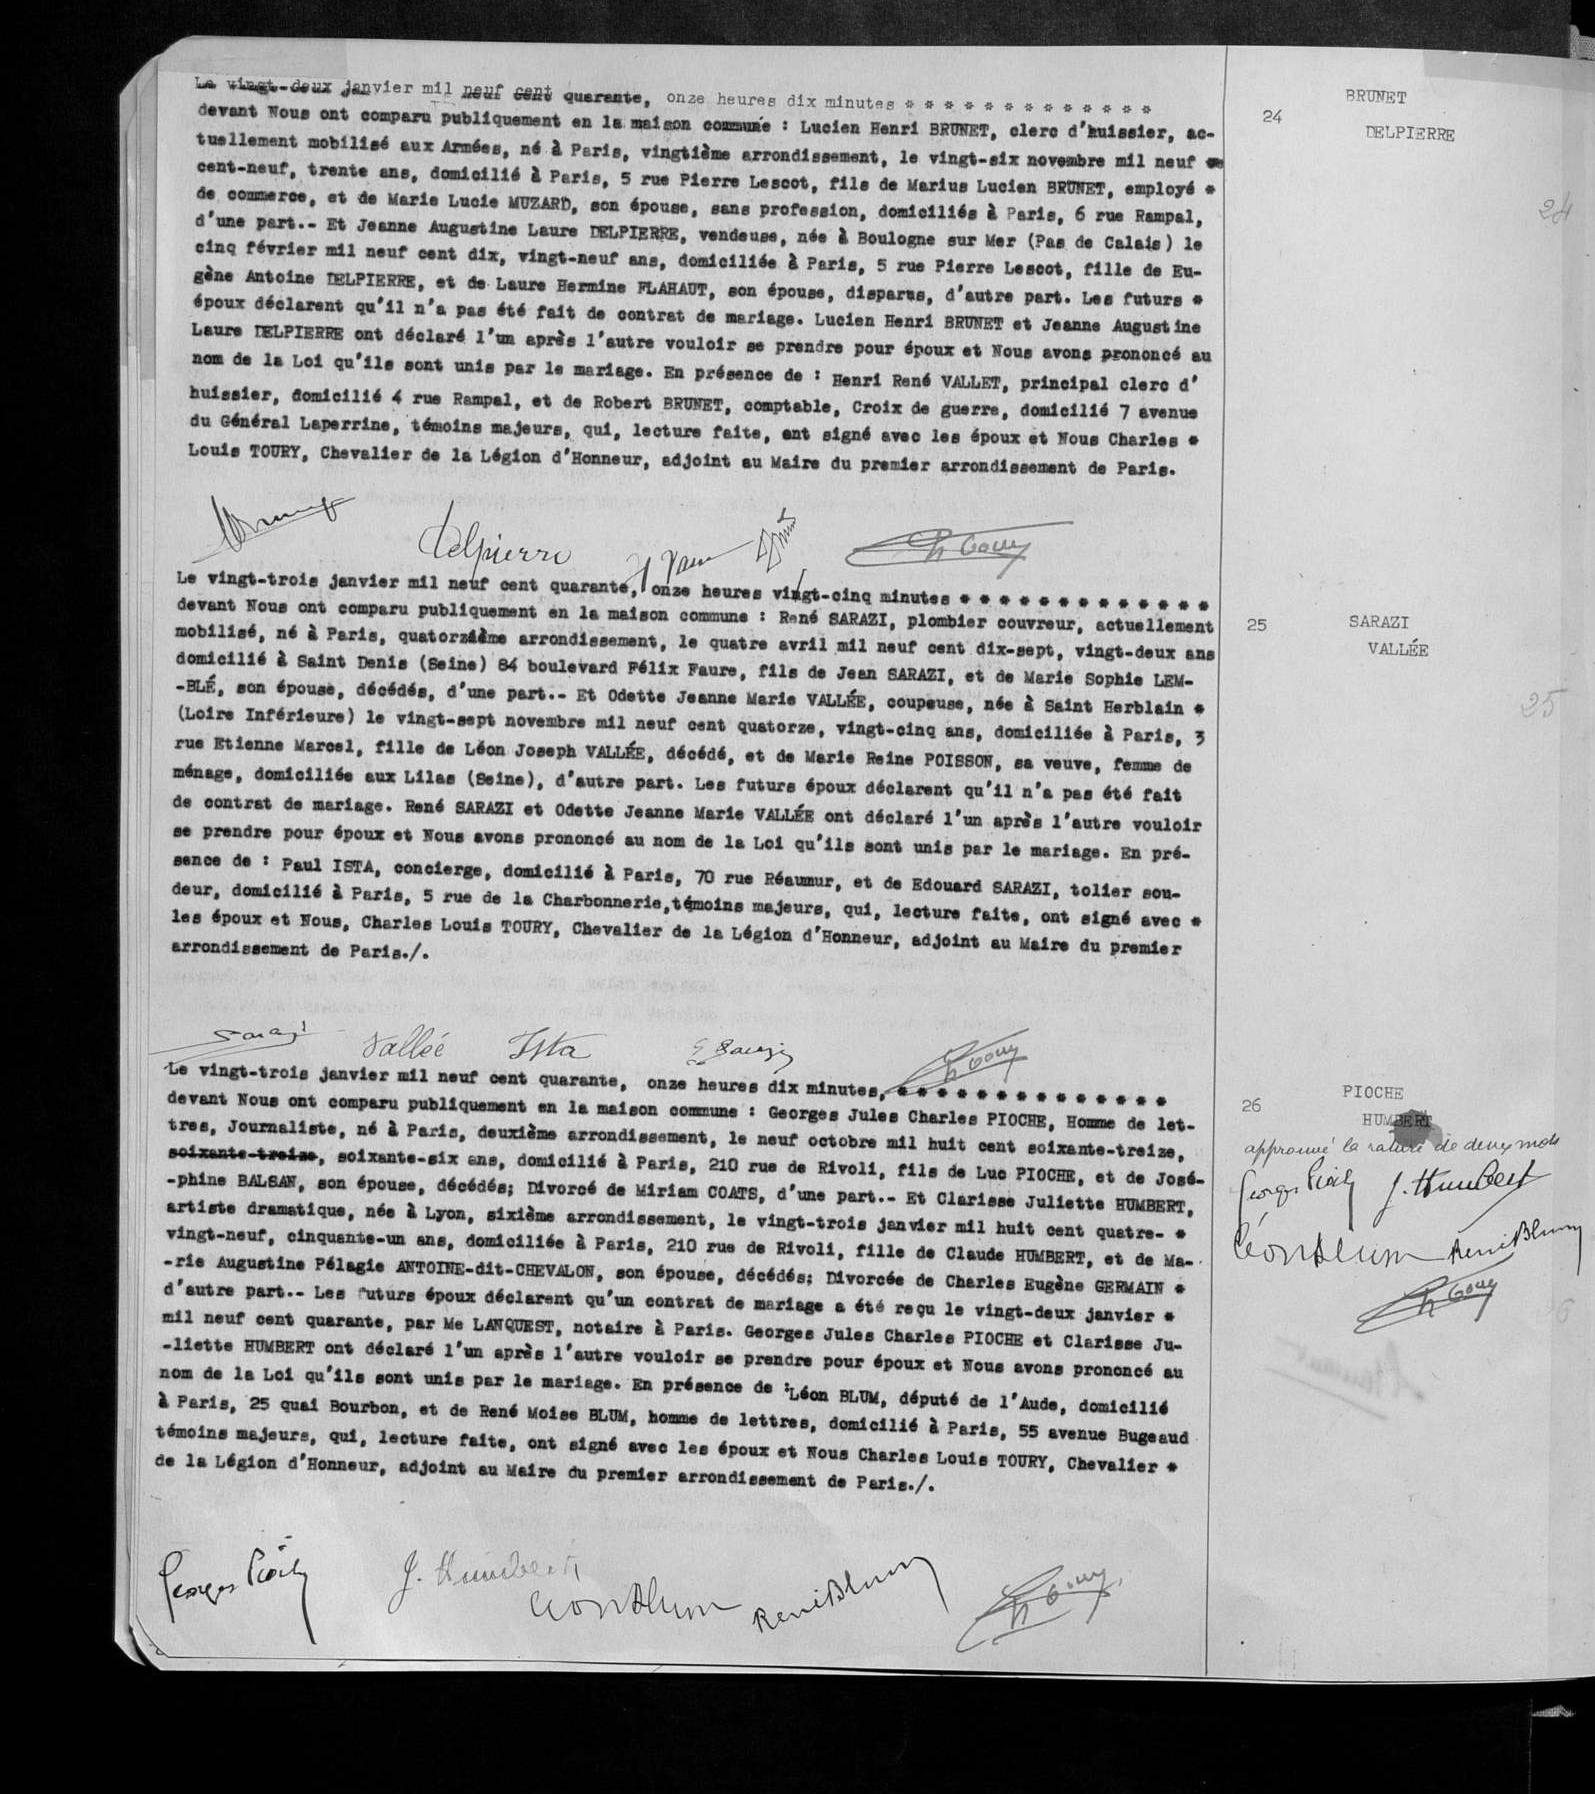Le vingt-deux janvier mil neuf cent quarante, onze heures dix minutes ****
devant Nous ont comparu publiquement en la maison commune: Lucien Henri BRUNET, clerc d'huissier, ac-
tuellement mobilisé aux Armées, né à Paris, vingtième arrondissement, le vingt-six novembre mil neuf *
cent-neuf, trente ans, domicilié à Paris, 5 rue Pierre Lescot, fils de Marius Lucien BRUNET, employé *
de commerce, et de Marie Lucie MUZARD, son épouse, sans profession, domiciliés à Paris, 6 rue Rampal,
d'une part .-Et Jeanne Augustine Laure DELPIERRE, vendeuse, née à Boulogne sur Mer (Pas de Calais) le
cinq février mil neuf cent dix, vingt-neuf ans, domiciliée à Paris, 5 rue Pierre Lescot, fille de Eu-
gène Antoine DELPIERRE, et de Laure Hermine FLAHAUT, son épouse disparus, d'autre part. Les futurs *
époux déclarent qu'il n'a pas été fait de contrat de mariage. Lucien Henri BRUNET et Jeanne Augustine
Laure DELPIERRE ont déclaré l'un après l'autre vouloir se prendre pour époux et Nous avons prononcé au
nom de la Loi qu'ils sont unis par le mariage. En présence de: Henri René VALLET, principal clerc d'
huissier, domicilié 4 rue Rampal, et de Robert BRUNET, comptable, Croix de guerre, domicilié 7 avenue
du Général Laperrine, témoins majeurs, qui, lecture faite, ont signé avec les époux et Nous Charles *
Louis TOURY, Chevalier de la Légion d'Honneur, adjoint au Marie du premier arrondissement de Paris.
Le vingt-trois janvier mil neuf cent quarante, onze heures vingt-cinq minutes ****
devant Nous ont comparu publiquement en la maison commune: René SARAZI, plombier couvreur, actuellement
mobilisé, né à Paris, quatorzième arrondissement, le quatre avril mil neuf cent dix-sept, vingt-deux ans
domicilié à Saint Denis (Seine) 84 boulevard Félix Faure, fils de Jean SARAZI, et de Marie Sophie LEM-
-BLE, son épouse, décédés, d'une part .-Et Odette Jeanne Marie VALLEE, coupeuse, née à Saint Herblain *
(Loire Inférieure) le vingt-sept novembre mil neuf cent quatorze, vingt-cinq ans, domiciliée à Paris, 3
rue Etienne Marcel, fille de Léon Jospeph VALLEE, décédé, et de Marie Reine POISSON, sa veuve, femme de
ménage, domiciliée aux Lilas (Seine), d'autre part. Les futurs époux déclarent qu'il n'a pas été fait
de contrat de mariage. René SARAZI et Odette Jeanne Maire VALLEE ont déclaré l'un après l'autre vouloir
se prendre pour époux et Nous avons prononcé au nom de la Loi qu'ils sont unis par le mariage. En pré-
sence de: Paul ISTA, concierge, domicilié à Paris, 70 rue Réaumur, et de Edouard SARAZI, tolier sou-
deur, domicilié à Paris, 5 rue de la Charbonnerie, témoins majeurs, qui, lecture faite, ont signé avec *
les époux et Nous, charles Louis TOURY, Chevalier de la légion d'Honneur, adjoint au Maire du premier
arrondissement de Paris ./.
Le vingt-trois janvier mil neuf cent quarante, onze heures dix minutes, ****
devant Nous ont comparu publiquement en la maison commune: Georges Jules Charles PIOCHE, Homme de let-
tres, Journaliste, né à Paris, deuxième arrondissement, le neuf octobre mil huit cent soixante-treize,
soixante-treize, soixante-six ans, domicilié à Paris, 210 rue de Rivoli, fils de Luc PIOCHE, et de José-
phine BALSAN, son épouse, décédés; Divorcé de Miriam COATS, d'une part .-Et Clarisse Juliette HUMBERT,
artiste dramatique, née à Lyon, sixième arrondissement, le vingt-trois janvier mil huit cent quatre -*
vingt-neuf, cinquante-un ans, domicilié à Paris, 210 rue de Rivoli, fille de Claude HUMBERT, et de Ma-
-rie Augustine Pélagie ANTOINE-dit-CHEVALON, son épouse, décédés; Divorcée de Charles Eugène GERMAIN *
d'autre part .-Les futurs époux déclarent qu'un contrat de mariage a été reçu le vingt-deux janvier *
mil neuf cent quarante, par Me LANQUEST, notaire à Paris. Georges Jules Charles PIOCHE et Clarisse Ju-
-liette HUMBERT ont déclaré l'un après l'autre vouloir se prendre pour époux et Nous avons prononcé au
nom de la Loi qu'ils sont unis par le mariage. En présence de Léon BLUM, député de l'Aude, domicilié
à Paris, 25 quai Bourbon, et de René Moise BLUM, homme de lettres, domicilié à Paris, 55 avenue Bugeaud
témoins majeurs, qui, lecture faite, ont signé avec les époux et Nous Charles Louis TOURY, Chevalier *
de la Légion d'Honneur, adjoint au Maire du premier arrondissement de Paris ./.
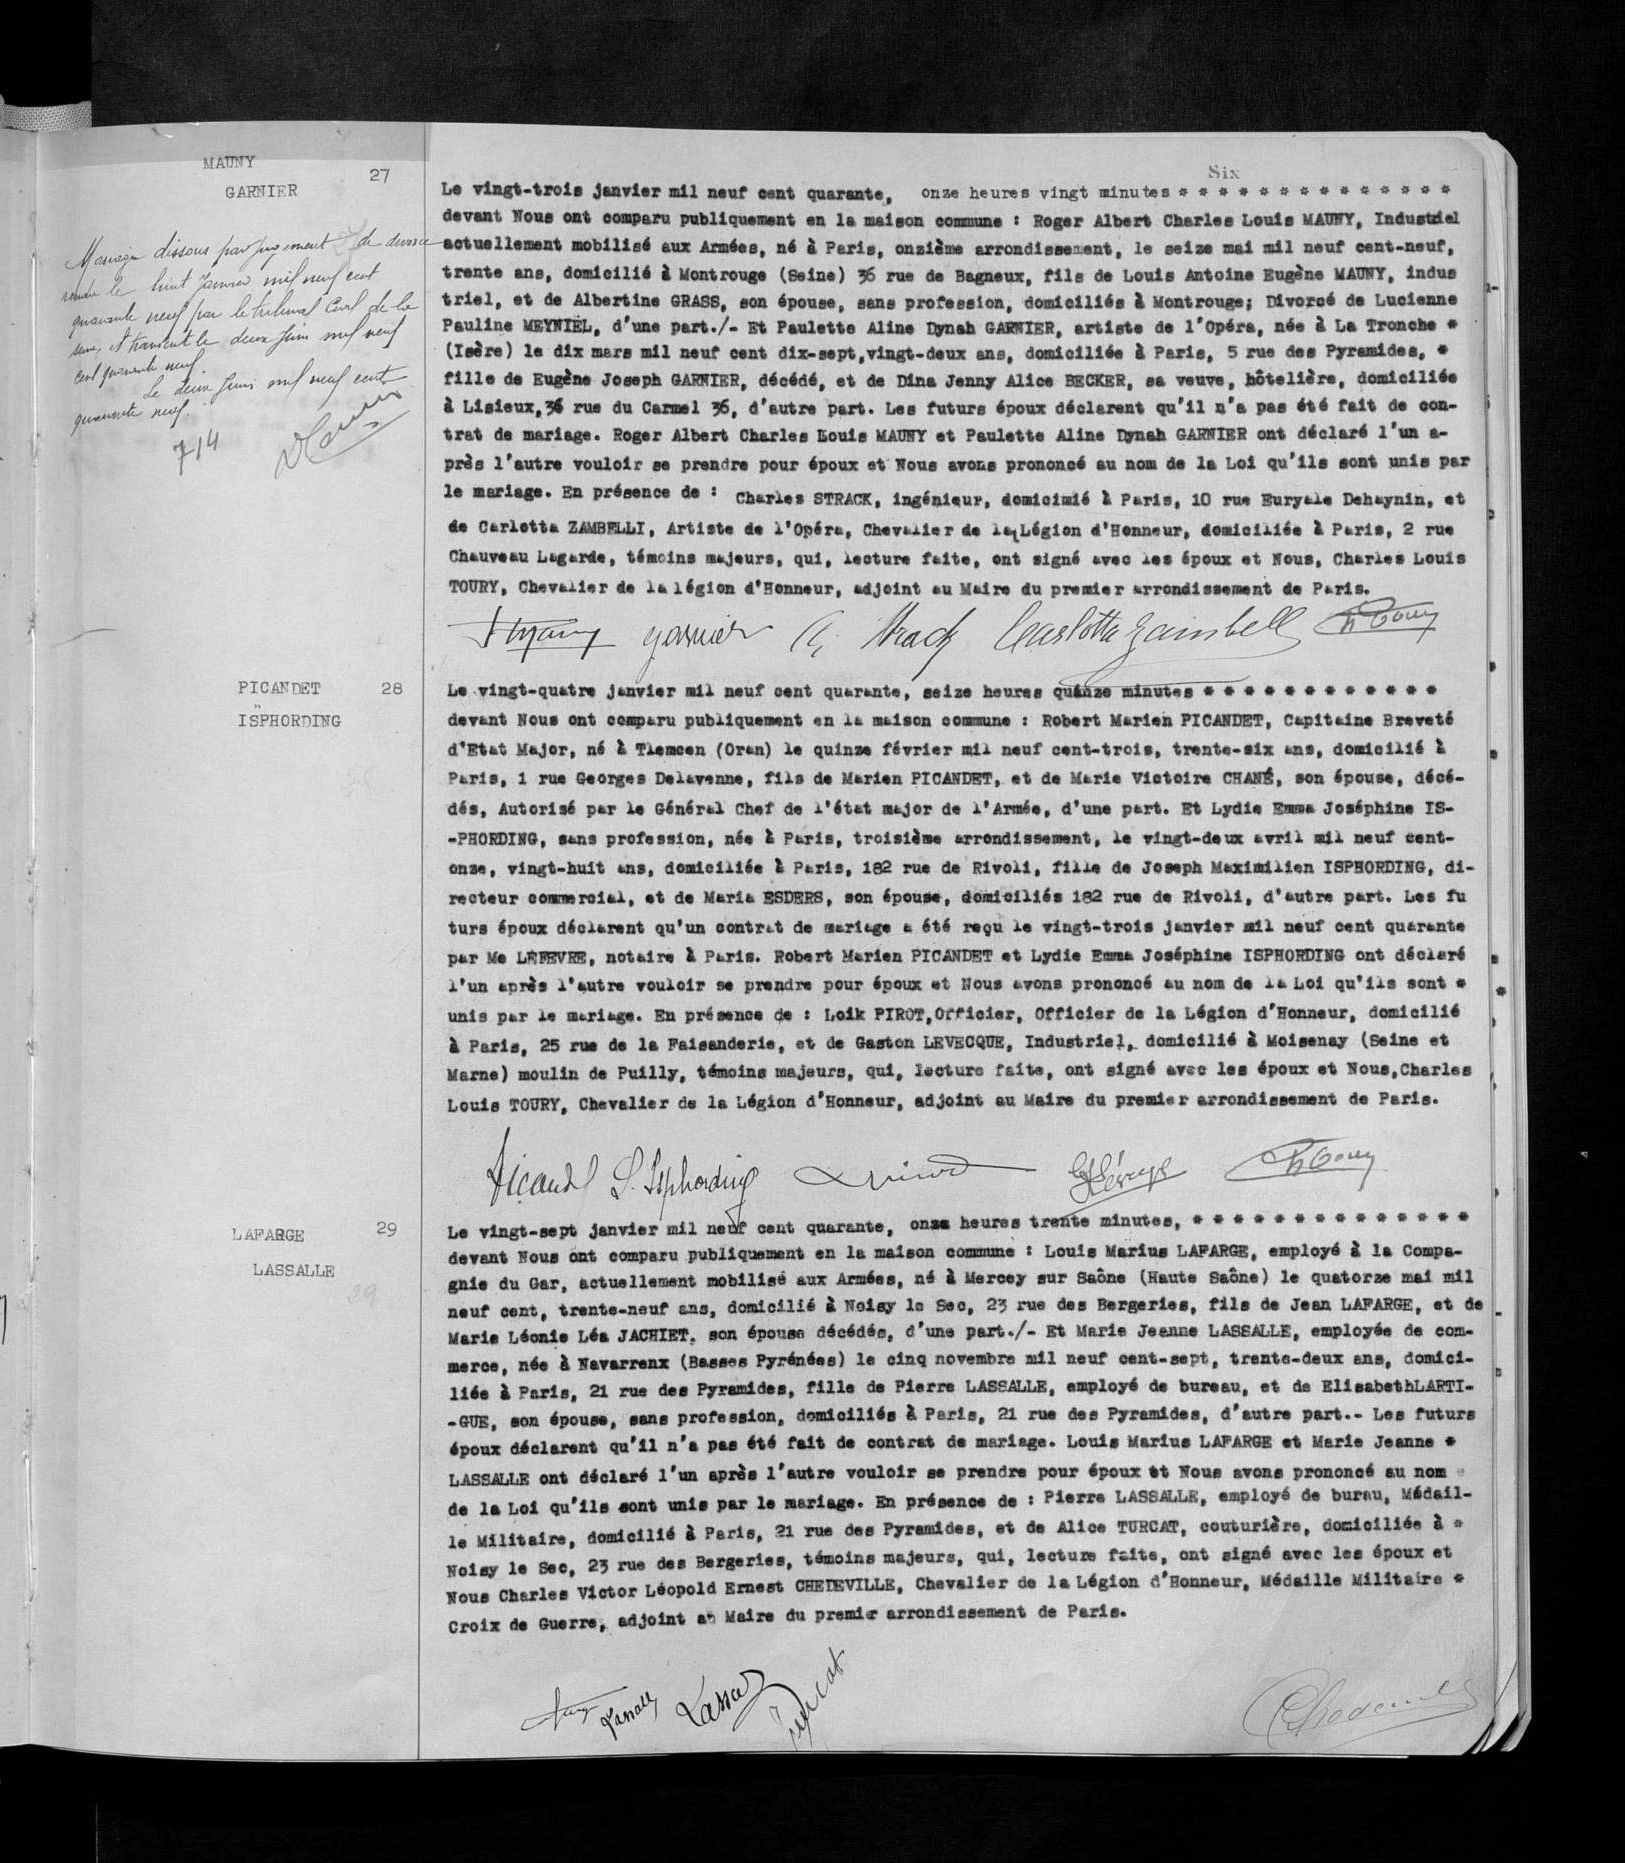Le vingt-trois janvier mil neuf cent quarante, onze heures vingt minutes ****
devant Nous ont comparu publiquement en la maison commune: Roger Albert Charles Louis MAUNY, Industriel
actuellement mobilisé aux Armées, né à Paris, onzième arrondissement, le seize mai mil neuf cent-neuf,
trente ans, domicilié à Montrouge (Seine) 36 rue de Bagneux, fils de Louis Antoine Eugène MAUNY, indus
triel, et de Albertine GRASS, son épouse, sans profession, domiciliés à Montrouge; Divorcé de Lucienne
Pauline MEYNEL, d'une part ./-Et Paulette Aline Dynah GARNIER, artiste de l'Opéra, née à La Tronche *
(Isère) le dix mars mil neuf cent dix-sept, vingt-deux ans, domiciliée à Paris, 5 rue des Pyramides, *
fille de Eugène Joseph GARNIER, décédé, et de Dina Jenny Alice BECKER, sa veuve, hôtelière, domiciliée
à Lisieux, 36 rue du Carmel 36, d'autre part. Les futurs époux déclarent qu'il n'a pas été fait de con-
trat de mariage. Roger Albert Charles Louis MAUNY et Paulette Aline Dynah GARNIER ont déclaré l'un a-
près l'autre vouloir se prendre pour époux et Nous avons prononcé au nom de la Loi qu'ils sont unis par
le mariage. En présence de: Charles STRACK, ingénieur, domicimié à Paris, 10 rue Euryale Dehaynin,
et de Carletta ZAMBELLI, Artiste de l'Opéra, Chevalier de Légion d'Honneur, domiciliée à Paris, 2 rue
Chauveau Lagarde, témoins majeurs, qui, lecture faite, ont signé avec les époux et Nous, Charles Louis
TOURY, Chevalier de la légion d'Honneur, adjoint au Mairee du premier arrondissement de Paris.
Le vingt-quatre janvier mil neuf cent quarante, seize heures quinze minutes ****
devant Nous ont comparu publiquement en la maison commune: Robert Marien PICANDET, Capitaine Breveté
d'Etat Major, né à Tiemcon (Oran) le quinze février mil neuf cent-trois, trente-six ans, domicilié à
Paris, 1 rue Georges Delavenne, fils de Marie PICANDET, et de Marie Victoire CHANE, son épouse, décé-
dés, Autorisé par le Général Chef de l'état major de l'armée, d'une part. Et Lydia Emma Joséphine IS-
-PHORDING, sans profession, née à Paris, troisième arrondissement, le vingt-deux avril mil neuf cent-
onze, vingt-huit ans, domiciliée à Paris, 182 rue de Rivoli, fille de Joseph Maximilien ISPHORDING, di-
recteur commercial, et de Maria ESDERS, son épouse, domiciliés, 182 rue de Rivoli, d'autre part. Les fu
turs époux déclarent qu'un contrat de mariage a été reçue vingt-trois janvier mil neuf cent quarante
par Me LEFEVRE, notaire à Paris. Robert Marien PICANDET et Lydie Emma Joséphine ISPHORDING ont déclaré
l'un après l'autre vouloir se prendre pour époux et Nous avons prononcé au nom de la Loi qu'ils sont *
unis par le mariage. En présence de: Loik PIROT, Officier, Officier de la Légion d'Honneur, domicilié
à Paris, 25 rue de la Faisanderie, et de Gaston LEVECQUE, Industriel, domicilié à Moisenay (Seine et
Marne) Moulin de Puilly, témoins majeurs, qui, lecture faite, ont signé avec les époux et Nous, Charles
Louis TOURY, Chevalier de la Légion d'Honneur, adjoint au Marie du premier arrondissement de Paris.
Le vingt-sept janvier mil neuf cent quarante, onze heures trente minutes ****
devant Nous ont comparu publiquement en la maison commune: Louis Marius LAFARGE, employé à la Compa-
gnie du Gar, actuellement mobilisé aux Armées, né à Mercey sur Saône (Haute Saône) le quatorze mai mil
neuf cent, trente-neuf ans, domicilié à Noisy le Sec, 23 rue des Bergeries, fils de Jean LAFARGE, et de
Marie Léonie Léa JACHIET, son épouse décédée, d'une part ./-Et marie Jeanne LASSALLE, employée de com-
merce, née à Navarrenx (Basses Pyrénées) le cinq novembre mil neuf cent-sept, trente-deux ans, domici-
liée à Paris, 21 rue des Pyramides, fille de Pierre LASSALLE, employé de bureau, et de Elisabeth LARTI-
-GUE, son épouse, sans profession, domiciliés à Paris, 21 rue des Pyramides, d'autre part .-Les futurs
époux déclarent qu'il n'a pas été fait de contrat de mariage. Louis Marius LAFARGE et Marie Jeanne *
LASSALLE ont déclaré l'un après l'autre vouloir se prendre pour époux et Nous avons prononcé au nom
de la Loi qu'ils sont unis par le mariage. En présence de Pierre LASSALLE, employé de burau, Médail-
le Militaire, domicilié à Paris, 21 rue des Pyramides, et de Alice TURCAT, couturière, domiciliée à *
Noisy le Sec, 23 rue des Bergeries, témoins majeurs, qui, lecture faite, ont signé avec les époux et
Nous Charles Victor Léopold Ernest CHELEVILLE, Chevalier de la Légion d'Honneur, Médaille Militaire *
Croix de Guerre, adjoint a Maire du premier arrondissement de Paris.